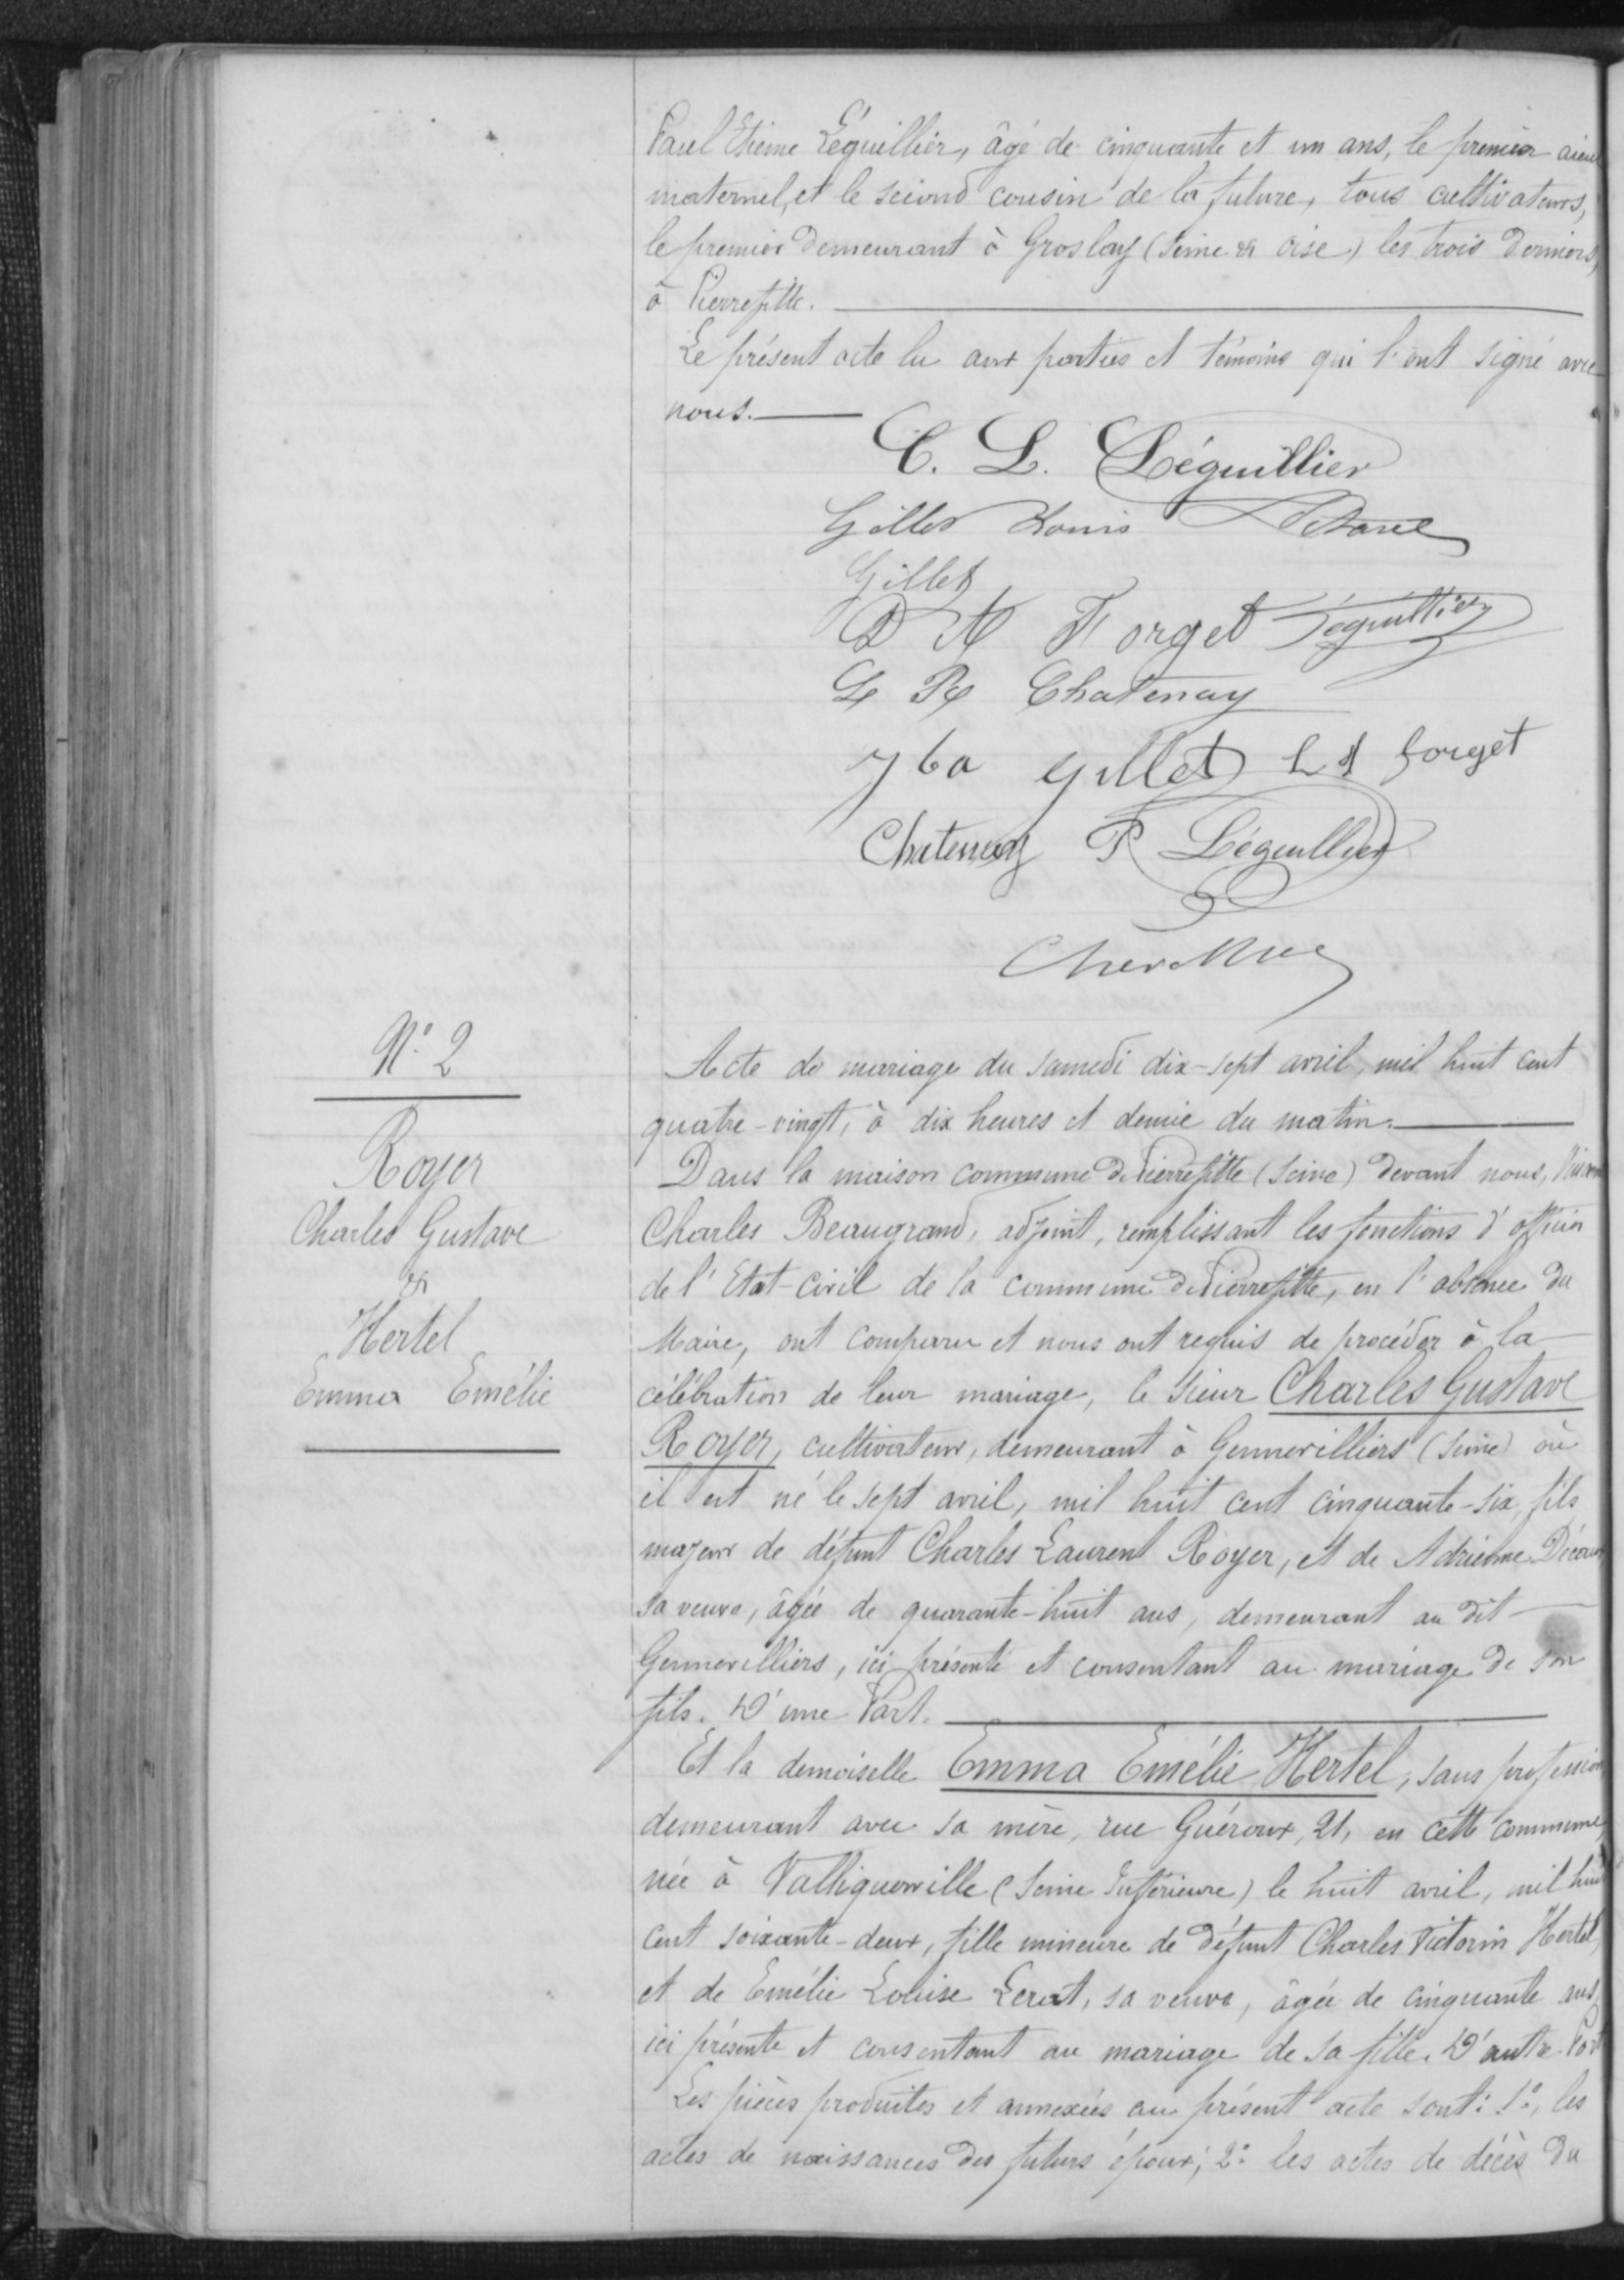Paul Etienne Léguillier, âgé de cinquante et un ans, le premier aieul
maternel, et le second cousin de la future, tous cultivateurs,
le premier demeurant à Groslay (Seine & Oise) les trois derniers,
à Pierfitte
Le présent acte lu aux partie set témoins qui l'ont signé avec
nous.
N°2
Royer
Charles Gustave
&
Hertel
Emma Emélie
Acte de mariage du samedi dix-sept avril mil huit cent
quatre-vingt, à dix heures et demie du matin.
Dans la maison commune de Pierrefitte (Seine) devant nous Vincent
Charles Beaugrand, adjoint, remplissant les fonctions d'officier
de l'Etat civil de la commune de Pierrefitte, en l'absence du
Maire, ont comparu et nous ont requis de procéder à la
célébration de leur mariage, le sieur Charles Gustave
Royer, cultivateur, demeurant à Gennevilliers (Seine) où
il est né le sept avril, mil huit cent cinquante-six, fils
majeur de défunt Charles Laurent Royer, et de Adrienne Déceveur
sa veuve, âgée de quarante-huit ans, demeuant au dix
Gennervilliers, ici présente et consentant au mariage de son
fils. D'une Part.
Et la demoiselle Emma Emelié Hertel, sans profession
demeurant avec sa mère, rue Guéroux, 21, en cette commune,
née à Valliguenille (Seine Inférieure) le huit avril, mil huit
cent soixante-deux, fille mineure de défunt Charles Victorin Hertel
et de Emélie Louise Lerat, sa veuve, âgée de cinquante ans
ici présente et consentant au mariage de sa fille. D'autre Part
Les pièces produites et annexées au présent acte senti 1°: les
actes de naissances des futurs époux; 2°-les actes de décès du
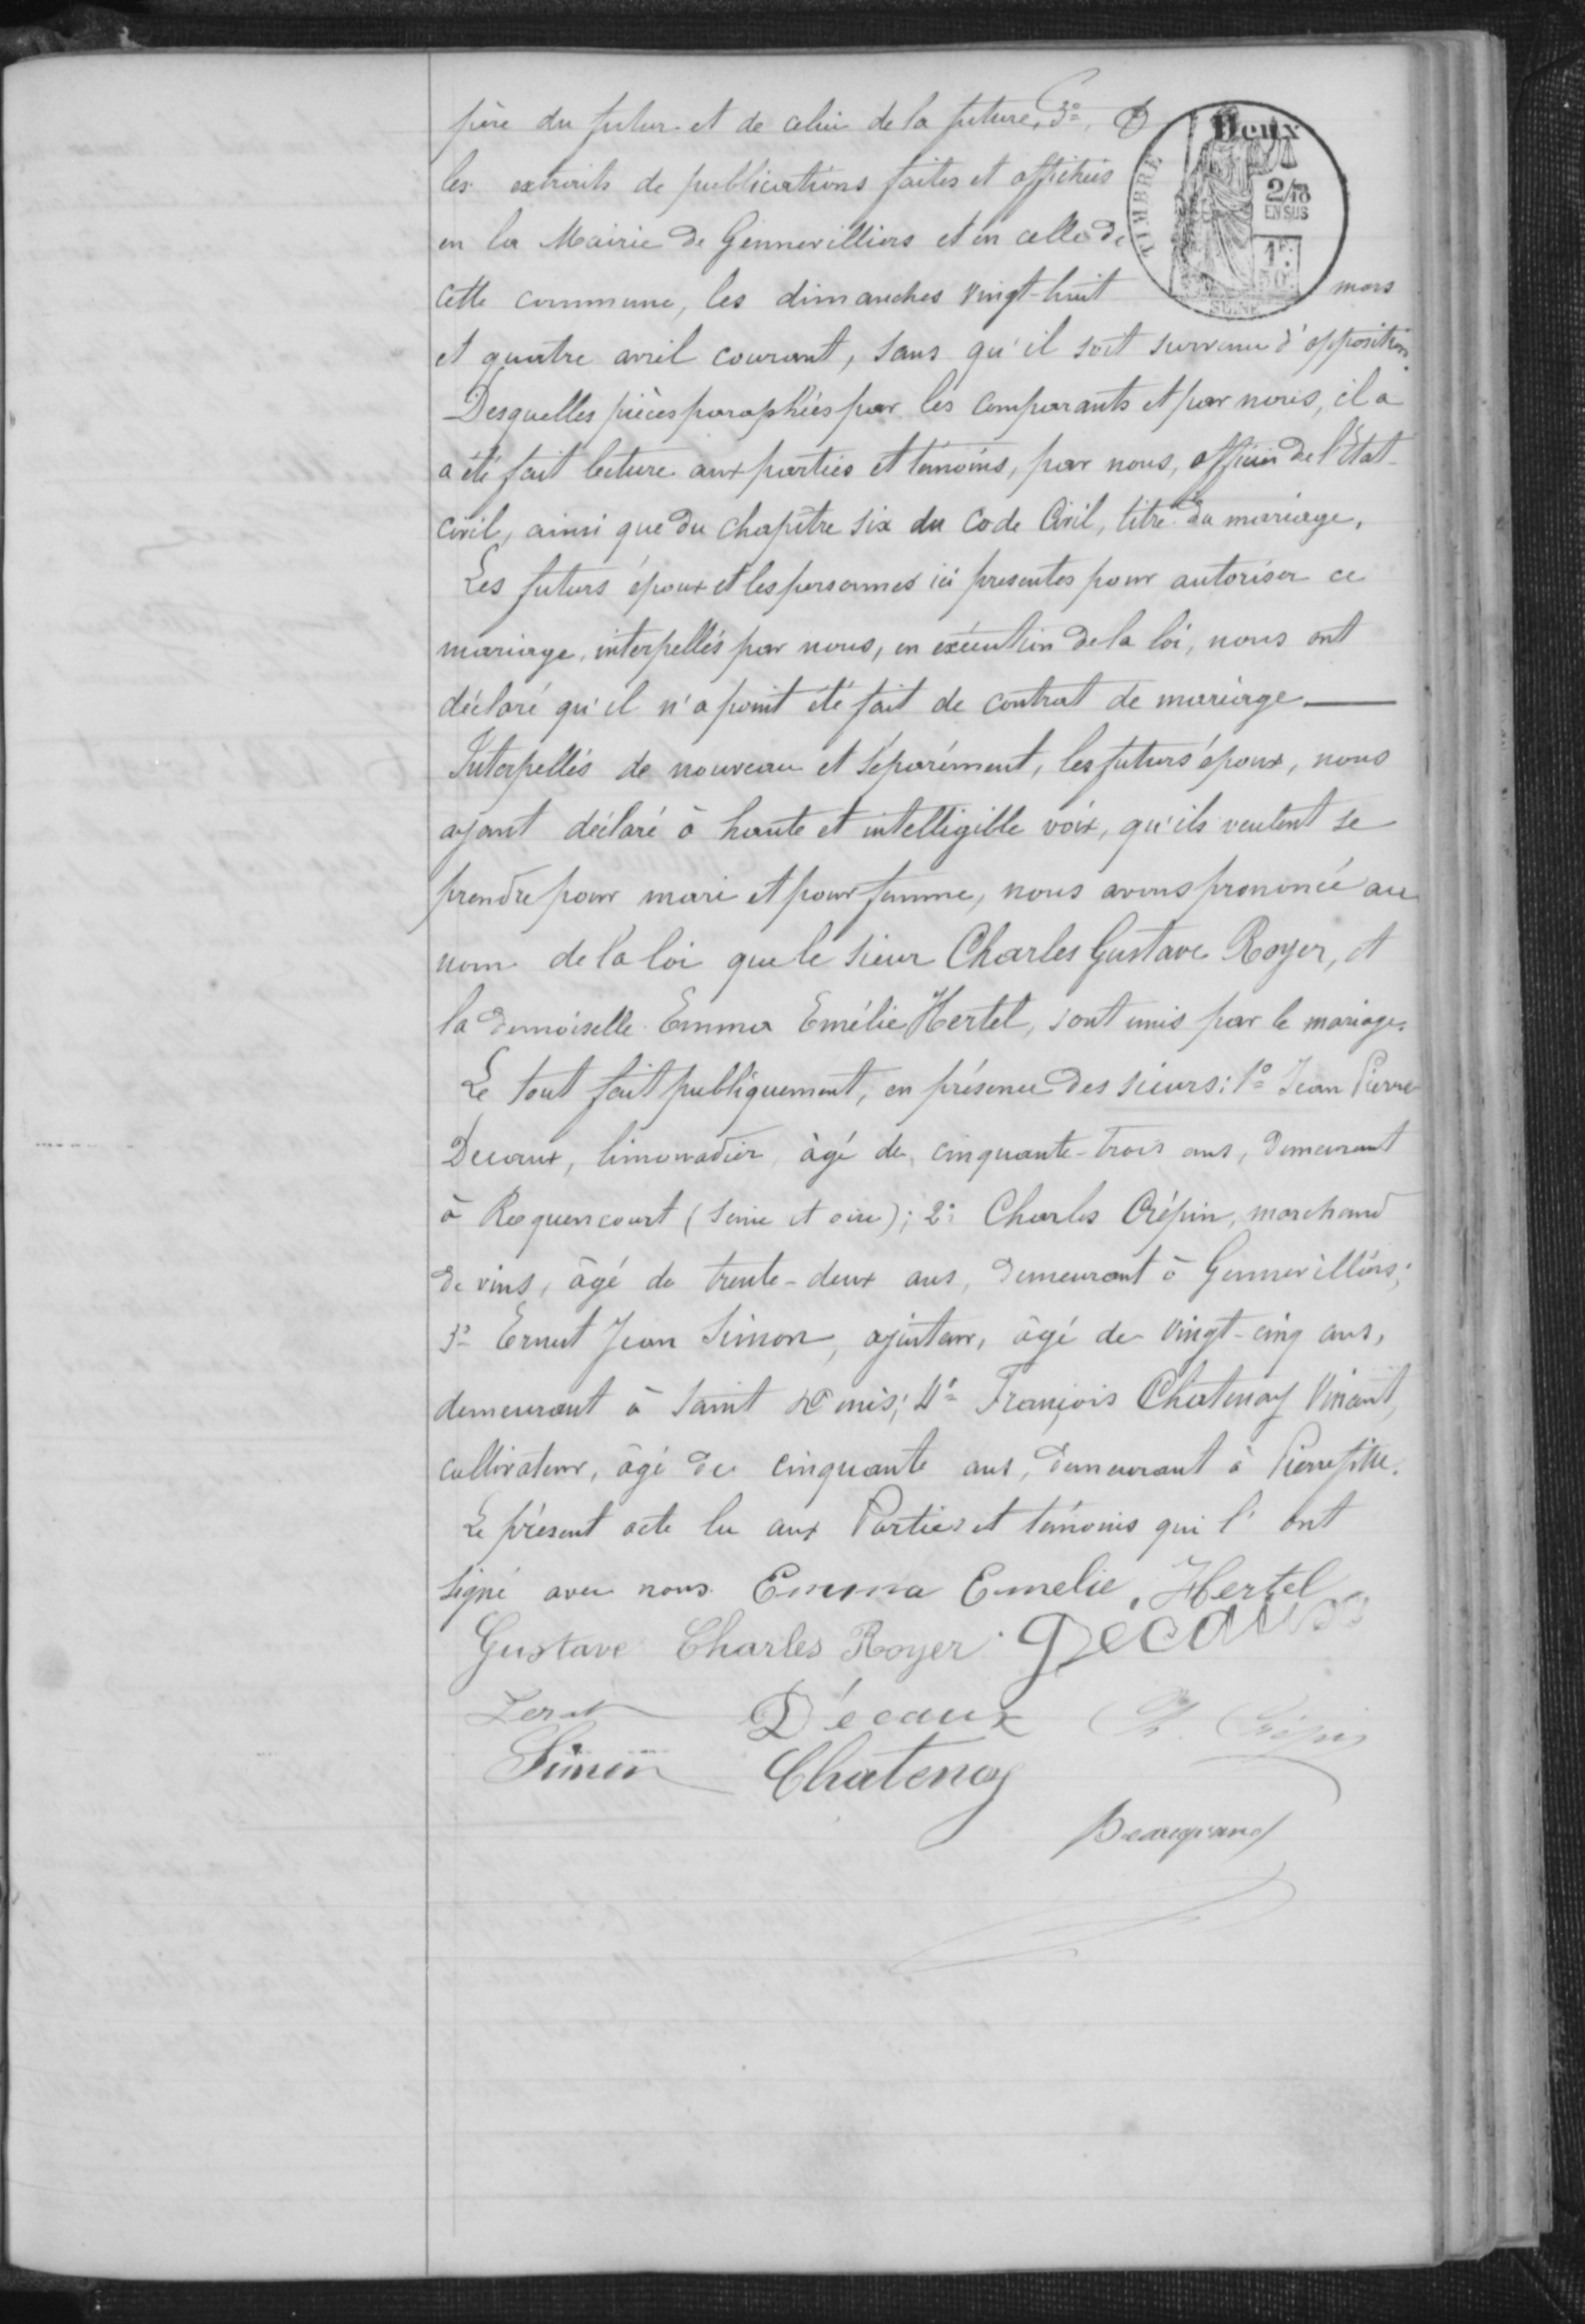frère du futur et de celui de la future, 3°:
les extraits de publications faites et affichées
en la Mairie de Gennevilliers et en celle de
cette commune, les dimanches vingt-huit mars
et quatre avril courant, sans qu'il soit survenue d'opposition
Desquelles pièces paraphées par les comparants et par nous, il a
a té fait lecture aux parties et témoins, par nous, officier de l'Etat
civil, ainsi que du chapitre six du code civil, titre du mariage.
Les futurs époux et les personnes ici présentes pour autoriser ce
mariage, interpellés par nous, en exécution de la loi, nous ont
déclaré qu'il n'a point été fait de contrat de mariage
Interpellés de nouveau et séparément, les futurs époux, nous
ayant déclaré à haute et intelligible voix, qu'ils veulent se
prendre pour mari et pour femme, nous avons prononcé au
nom de la loi que le sieur Charles Gustave Royer, et
la demoiselle Emma Emélie Hertel, sont unis par le mariage
Le tout fait publiquement, en présence des sieurs: 1°/ Jean Pierre
Decant, limonadier, âgé de cinquante-trois ans, demeurant
à Roquencourt (Seine et Oise); 2°/ Charles crépin, marchand
de vins, âgé de trente-deux ans, demeurant à Gennevilliers;
3°-Ernest Jean Simon, ajusteur, âgé de vingt-cinq ans,
demeurant à Saint Denis; 4°/ François Chastenay Viviant,
cultivateur, âgé de cinquante ans, demeurant à Pierrefitte.
Le présent acte lu aux parties et témoins qui l'ont
signé avec nous Emma Emelie, Hertel,
Gustave Charles Royer Décaux